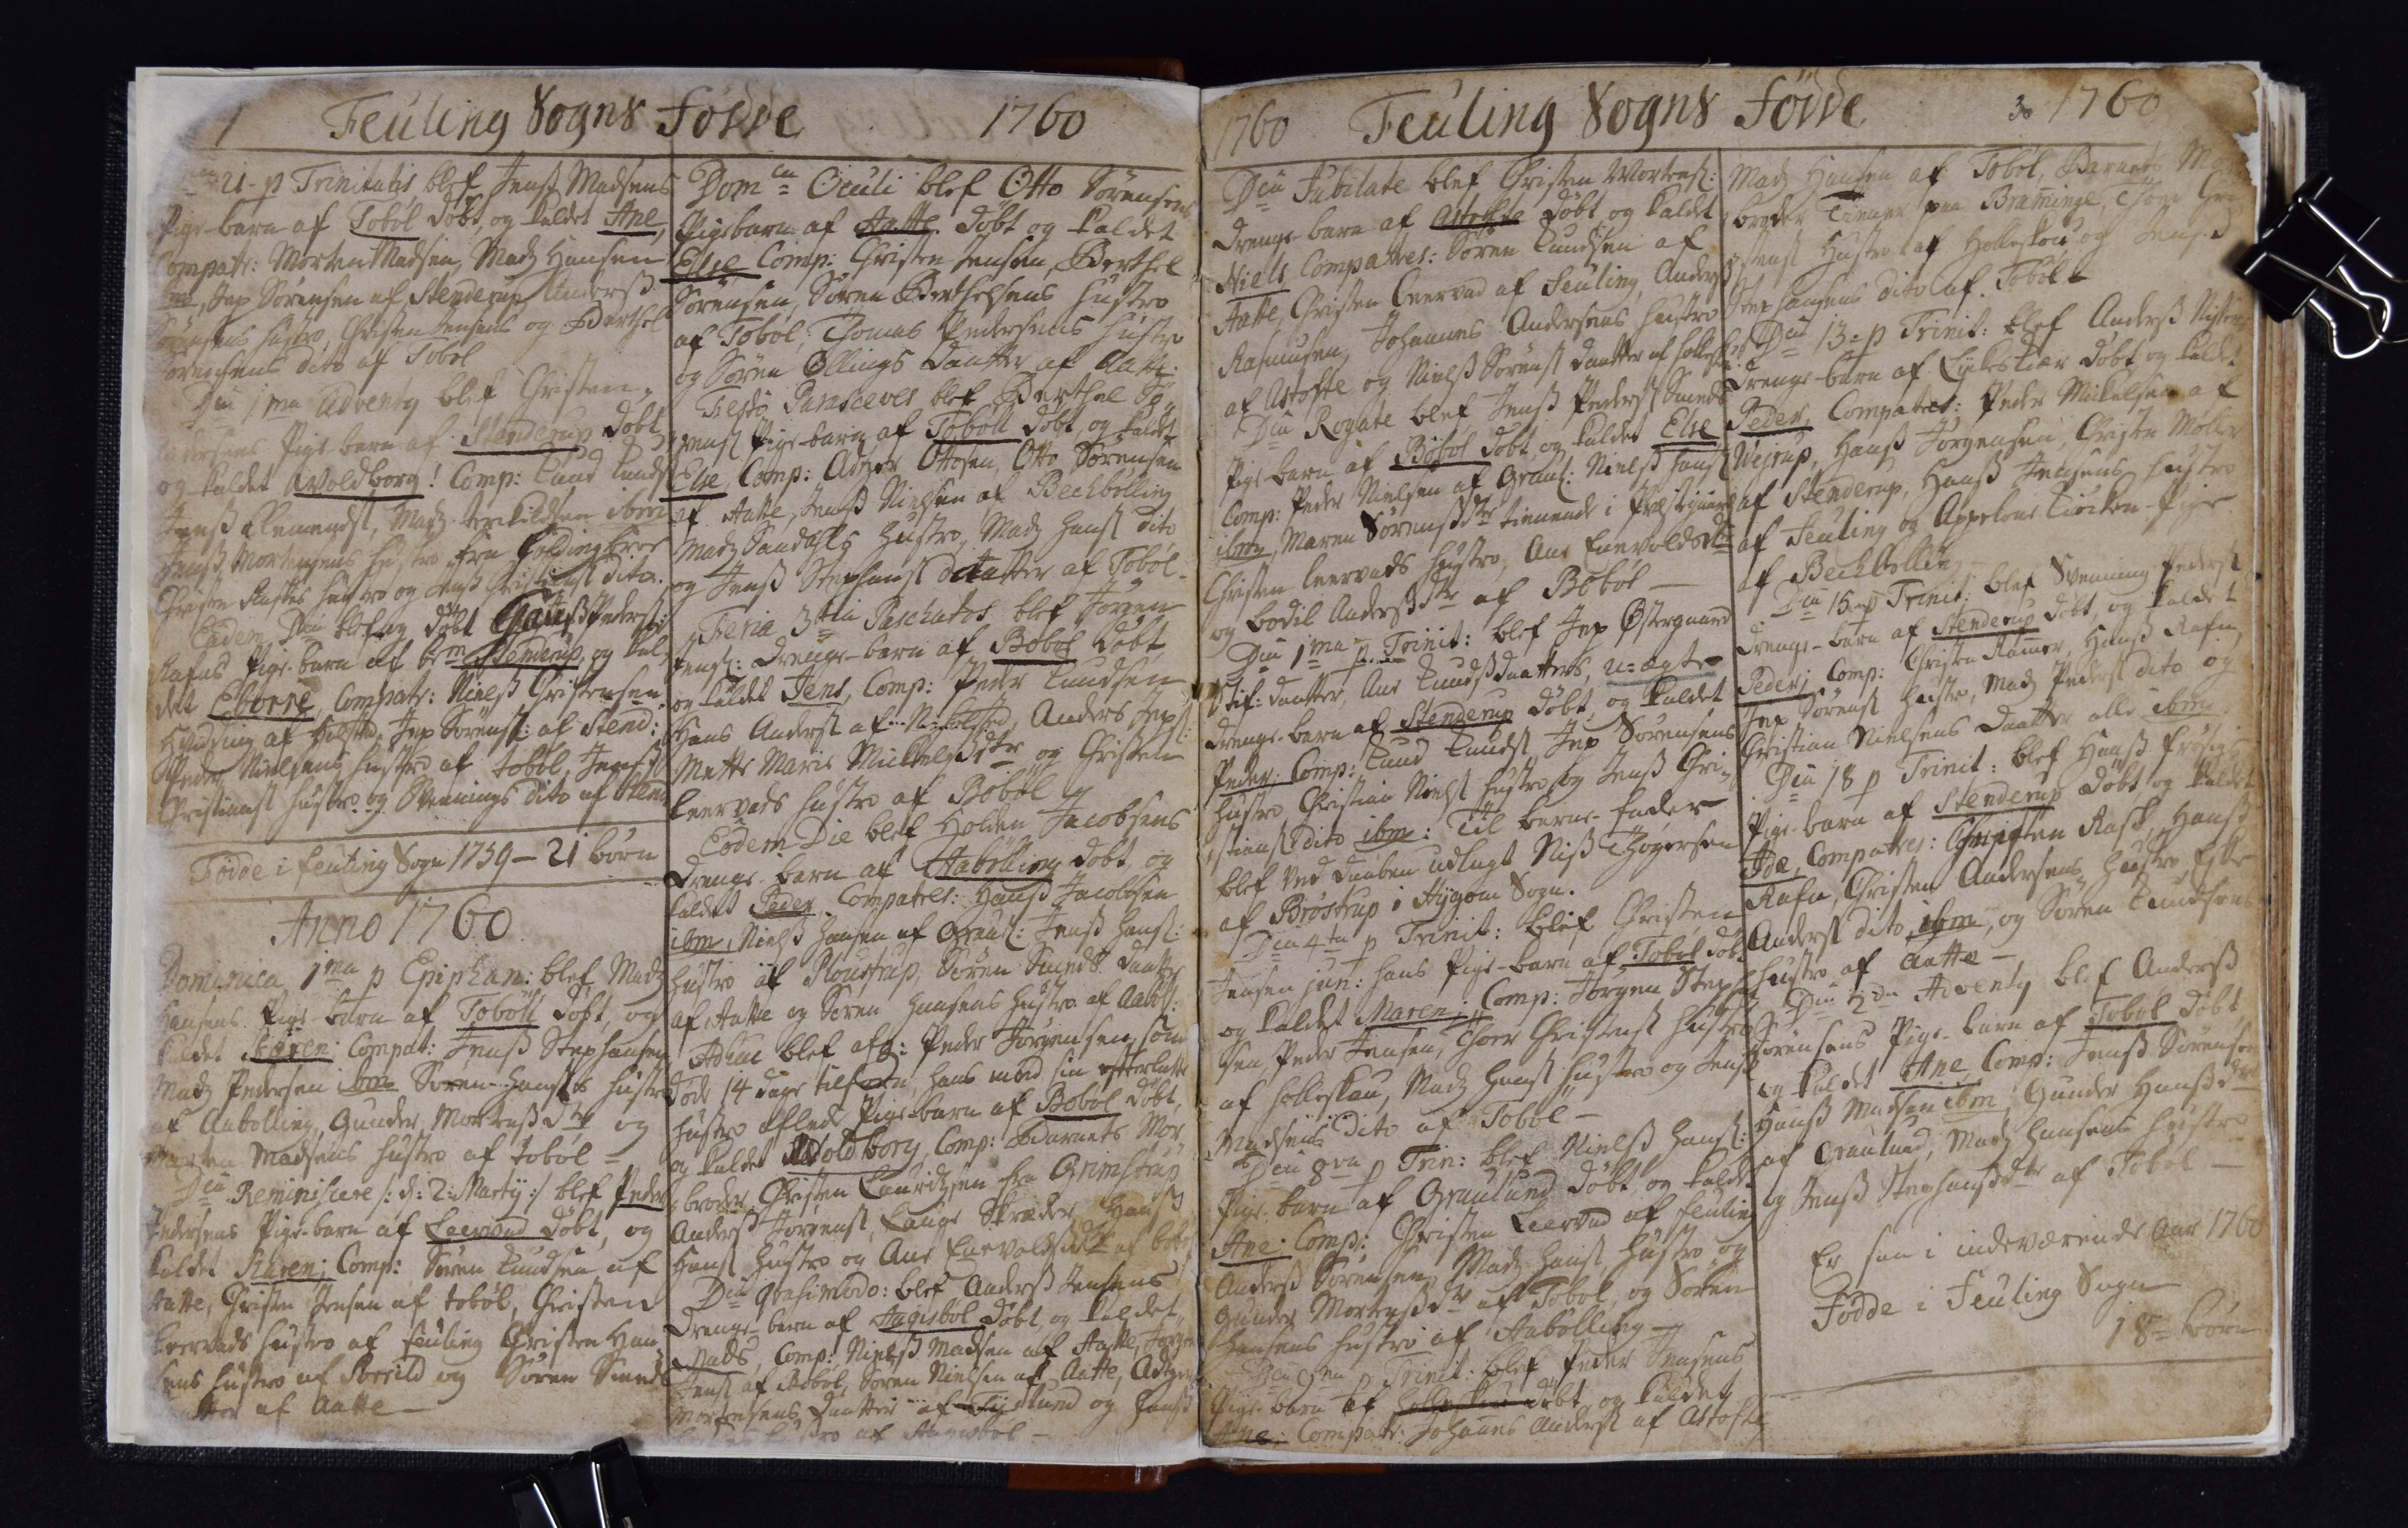Feulings Sogns Fødde
Dca 21. p Trinitatis blef Jens Madsens
Pige-barn af Tobøl døbt og kaldet Ane
Compatr: Morten Madsen, Madz Hansen
##, Jap Sørensen af Stenderup Anderss
Sørensens Hustro, Christen Jensens og Berthel
Sørensens dito af Tobøl
Dca 1ma Advent## blef Christen
##dersens Pige-barn af Stenderup døbt
og kaldet Woldborg! Comp: Knud Lunds
Hans Clemmeds, Madz Terkildsen ibm
Jenss Mortensens Hustro fra Coldinbroe
Christen Raskes Hustro og Jens Christias dito.
Eôdem Dca blef og døbt Clauss Peders:
Rafes Pige-barn af bem Stenderup, og kal¬
det Eborre Compatr: Niels Christensen
Hvidding af Holsted, Jep Sørens af Stend:
Peder Nielsens Hustro af Tobøl, Jens
Christias Hustro og Svennings dito af Stend
Fødde i Feuting Sogn 1759 - 21 børn
Anno 1760
Dominica 1ma p Epiphan: blef Madz
Hansens Pige-barn af Tobøll døbt, og
kaldet Karen Compat: Jens Stephansen
Madz Pedersen ibid Søren Hanss Hustro
af Aabølling, Gunder Mortenssdtr og
## Madsens Hustro af Tobøl
Dca Reminiscere /: d: 2 Martij :/ blef Peder
Pedersens Pige-barn af Leervad døbt, og
kaldet Karen Comp: Søren Knudsen af
Aatte Christen Jensen af Tobøl, Christen
Leervads Hustro af Feuling Christen Han¬
sens Hustro af Sorrild og Søren Smeds
Datter af Aatte
1760
Dom ca Oculi bleff Otto Sørensens
Pigebarn af Aatte døbt og kaldet
Else Comp: Christen Jensen Berthel
Sørensen, Søren Bertelsens Hustro
af Tobøl, Thomas Pedersens Hustro
og Søren Ollings Daatter af Aatte.
Festo Purasceves blef Berthel Sø¬
rens Pigebarn af Tobølt døbt, og kaldet
Else Comp: Adtzer Ottosen, Otte Sørensen
af Aatte, Jens Nielsøn af Bechbølling
Madz Sandahls Hustro Madz Hans dito
og Jens Stephans Daatter af Tobøl.
Feria 3tia Paschatos blef Jørgen
Jens: Drenge-barn af Bobøl døbt
og kaldet Jens, Comp: Peder Knudsen
Hans Andersen af N Holsted, Anders Jeps:
Mette Marie Mickelsdtr og Christen
Leervars Hustro af Bobøl
Eodem Die blef Holden Jacobsens
Drenge-barn af Aabølling døbt, og
kaldet Peder Compatres: Hans Jacobsen
ibm, Niels Hansen af Grau: Jens Hans:
Hustro af Ploustrup, Søren Smeds Daatter
af Aatte og Søren Hansens Hustru af Aabøl:
Ad## blef afg: Peder Jørgensen som
døde 14 Dage tilforn hands med sin efterlatte
Hustro afled Pige-barn af Bobøl døbt,
og kaldet Voldborg, Comp: Barnets Mor¬
broder Christen Lauridtzen fra Grimstrup
Anders Jørgens, Lauge Skræder, Hanss
Hans Hustro og Ane Enevoldssdtr af bobøl
Dca Qvasimod: blef Anders Jensens
Drenge-barn af Angisbøl døbt, og kaldet
Mads, Comp: Nielss Madsen af Aatte, Jørgen
Jens af Bobøl, Søren Nielsøn af Aatte, Adt##
Mortensens Daatter af Tiislund og Hans
## Hustro af Angisbøl
1760
Feuling Sogns Fødde
Dca Jubilate blef Christen Mortens¬
Drenge-barn af Astofte døbt og kaldet
Niels Compattes: Søren Knudsen af
Aatte, Christen Leervad af Feuling, Anders
Rasmusen Johannes Andersens Hustro
af Astofte og Niels Sørens Daatter af Holleskou
Dca Rogate blef Jenss Peders Smeds
Pige Barn af Bobøl døbt, og kaldet Else
Comp: Peder Nielsen af Graul: Niels Hans
ibm Maren Sørenssdtr tienende i Præstegaard
Christen Leervads Hustro, Ane Enevoldsdtr
og Bodil Andersdtr af Bobøl
Dom 1ma p Trinit: blef Jep Østergaard
Stif=datter, Ane Knudssdaatters u=ægte
Drenge-barn af Stenderup døbt, og kaldet
Peder; Comp:: Knud Kunds Jep Sørensens
Hustro, Christian Niels Hustro og Jens Chri¬
stians dito ibm: Til Barne-Fader
blef ved Daaben udlagt Niss Thøgersen
af Brøstrup i Hygom Sogn.
Dca 4ta p Trinit: blef Christen
Jensen Jun: hans Pige-barn af Tobøl døbt
og kaldet Maren; Comp: Jørgen Stepha
sen, Peder Jensen, Thoer Christens Hustro
af holleskou, Madz Hans Hustro og Jens
Madsens dito af Tobøl
Dca 8va p Trin: blef Nielss Hans
Pige-Barn af Graulund døbt og kaldet
Ane; Comp: Christen Leervad af JFeuling
Anders Sørensen, Madz Hans Hustro og
Gunder Mortenssdtr af Tobøl, og Søren
Hansens Hustro af Aabølling
Dca 9na p Trinit: blef Peder Jensens
Pige barn af Holleskou døbt og kaldet
Ane; Compatr: Johannes Anders af Astofte
30
1760
Madz Hansen af Tobøl Barnet Mor##
broder Tiener paa Bramminge, Thoer Chri¬
sens Hustro af Holleskou og Jenss
Stephansens dito af Tobøl
Dca 13 p Trinit: blef Anders Nissens
Drenge-barn af Lykeskiær døbt og kaldet
Peder Compatres: Peder Mickelsøn af
Wejrup, Hans Jørgensen, Christen Møller
af Stenderup, Hans Jensens Hustro
af Feuling og Appelone Kirke-Pige
af Bechbølling
Dca 15## p Trinit: blef Svenning Peders
Drenge-barn af Stenderup døbt, og kaldet
Peder; Comp: Christen Rammer, Hans Rafn,
Jep Sørens Hustr, Madz Peders dito og
Christian Nielsens Daatter alle ibm
Dca 18 p Trinit: blef Hanss Frøsigs
Pige-barn af Stenderup døbt, og kaldet
Ide, Compatres: Christen Rask, Hans
Rafn, Christen Andersens Hustroi, Eske
Anders dito, ibm, og Søren Knudsøns
Hustro af Aatte-
Dca 2den Advent## blev Anderss
Sørnsens Pige-barn af Tobøl døbt
og kaldet Ane, Comp: Jenss Sørensen
Hanss Madsen ibm, Gunder Hanssdtr
af Graulund, Madz Hansens Hustro
og Jens Stephansdtr af Tobøl.
Er saa i indeværende Aar 1760
Fødde i Feuling Sogn
18## Børn